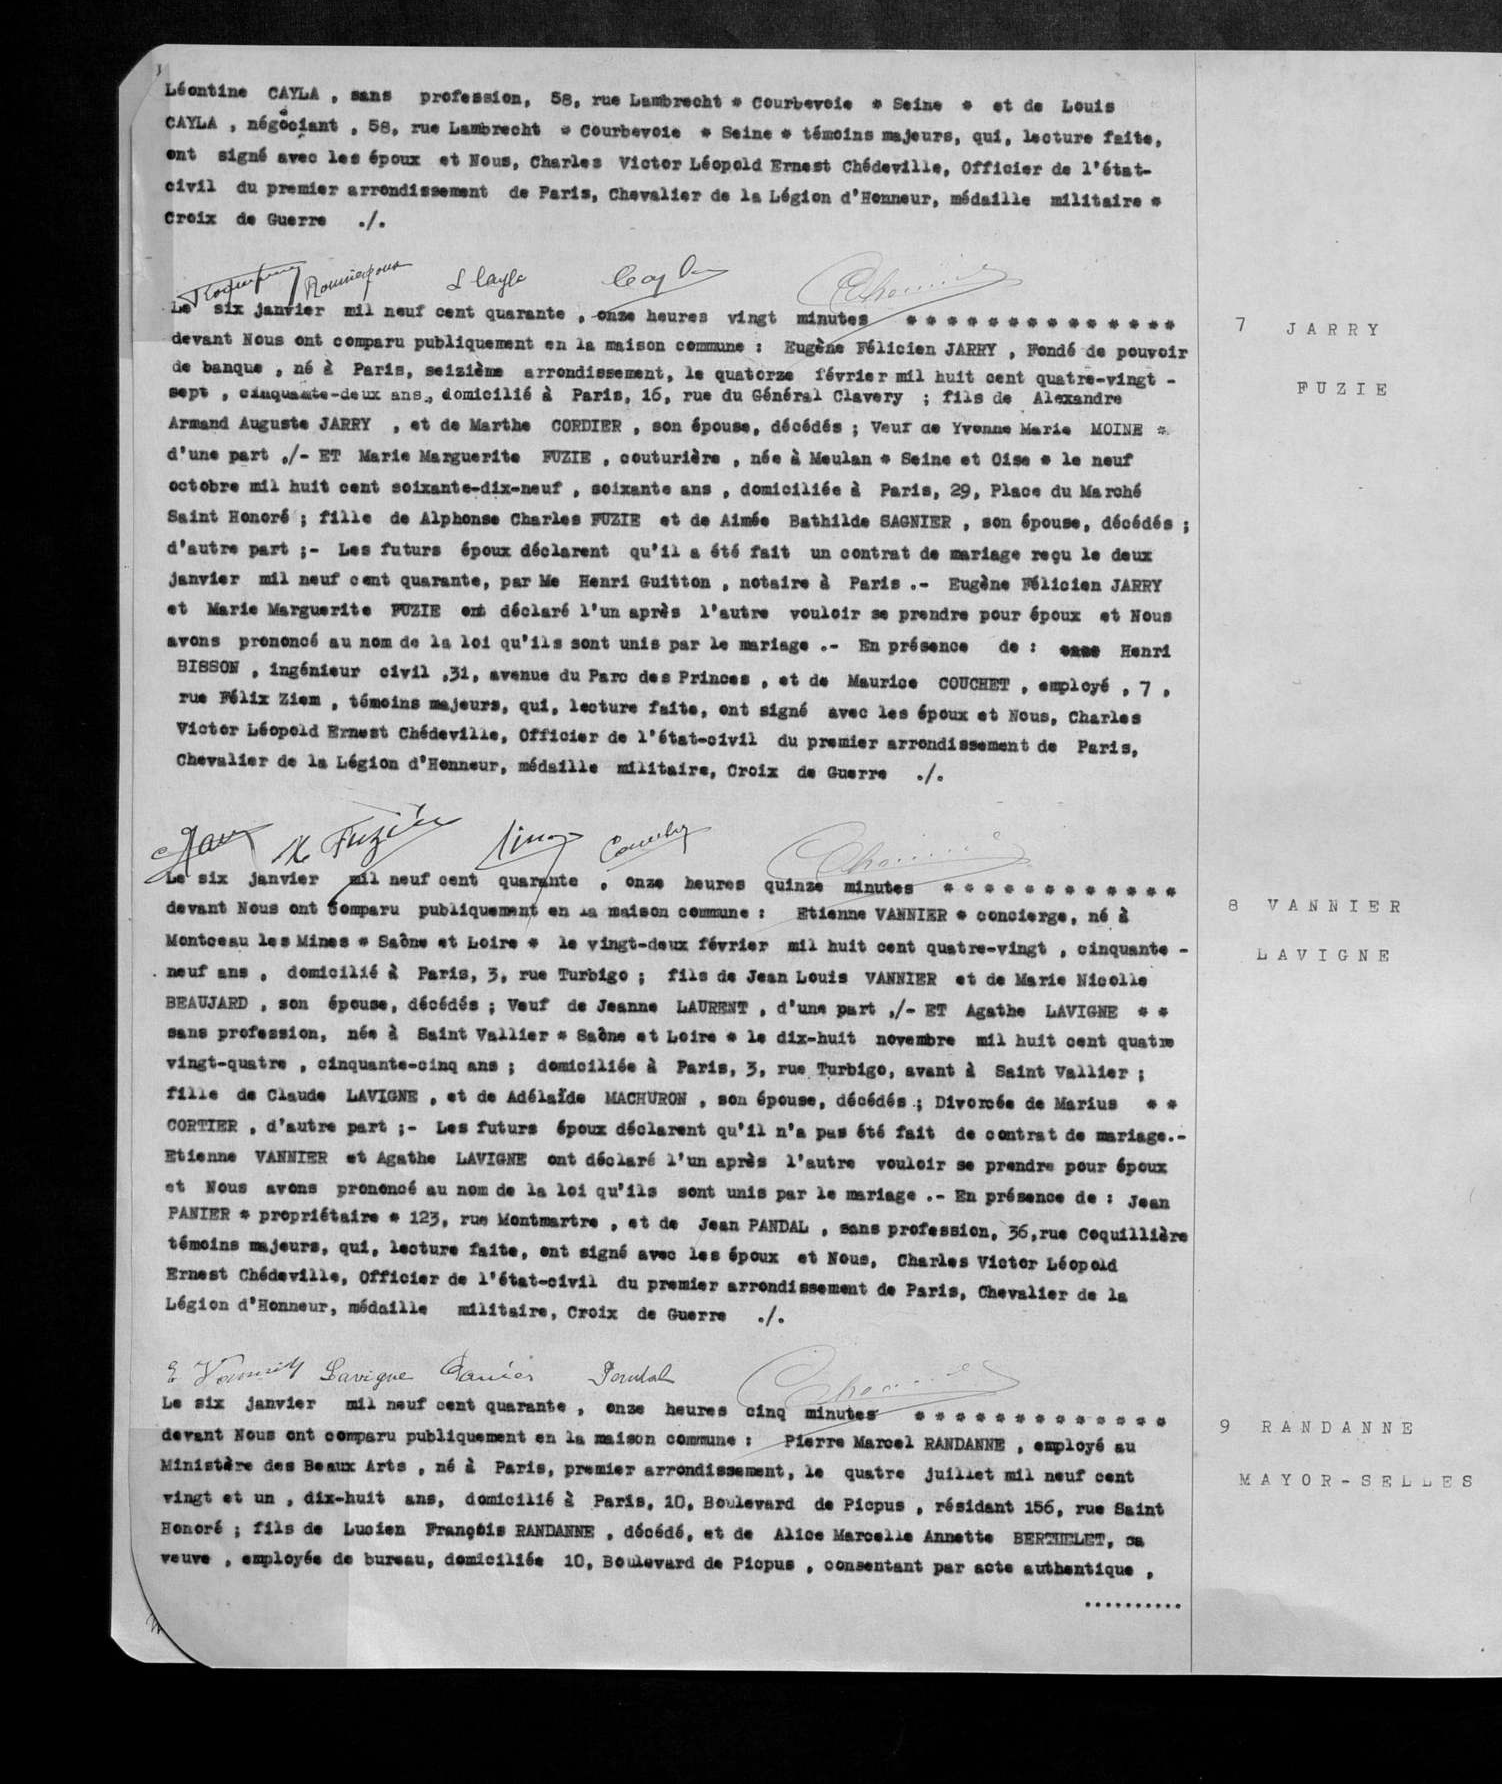Léontine CAYLA, sans profession, 58, rue Lambrecht * Courbevoie * Seine * et de Louis
CAYLA, négociant, 58, rue Lambrecht * Courbevoie * Seine * témoins majeurs, qui, lecture faite,
ont signé avec les époux et Nous, Charles Victor Léopolf Ernest Chédeville, Officier de l'état
civil du premier arrondissement de Paris, Chevalier de la Légion d'Honneur, médaille militaire *
Croix de Guerre ./.
Le six janvier mil neuf cent quarante, onze heures vingt minutes ****
devant Nous ont comparu publiquement en la maison commune: Eugène Félicien JARRY, Fondé de pouvoir
de banque, né à Paris, seizième arrondissement, le quatorze février mil huit cent quatre-vingt
sept, cinquante-deux ans, domicilié à Paris, 16 rue du Général Clavery; fils de Alexandre
Armand Auguste JARRY, et de Marthe CORDIER, son épouse, décédés; Veuf de Yvonne Marie MOINE *
d'une part ,/-ET Marie Marguerite FUZIE, couturière, née à Meulan * Seine et Oise * le neuf
octobre mil huit cent soixante-dix-neuf, soixante ans, domiciliée à Paris, 29, Place du Marché
Saint Honoré; fille de Alphone Charles FUZIE et de aimée Mathilde SAGNIER, son épouse, décédés;
d'autre part ;-Les futurs épouses déclarent qu'il a été fait un contrat de mariage reçu le deux
janvier mil neuf cent quarante, par Me Henri Guitton, notaire à Paris .-Eugène Félicien JARRY
et Marie Marguerite FUZIE ont déclaré l'un après l'autre vouloir se prendre pour époux et Nous
avons prononcé au nom de la loi qu'ils sont unis par le mariage .-En présence de: **** Henri
BISSON, ingénieur civil, 31 avenue du Parc des Princes, et de Maurice COUCHET, employé,
7, rue Félix Ziem, témoins majeurs, qui, lecture faite, ont signé avec les époux et Nous, Charles
Victor Léopold Ernest Chédeville, Officier de l'état-civil du premier arrondissement de Paris,
Chevalier de la Légion d'Honneur, médaille militaire, Croix de Guerre ./.
Le six janvier mil neuf cent quarante, onze heures quinze minutes ****
devant Nous ont comparu publiquement en la maison commune: Etienne WANNIER * concierge, né à
Montceau les Mines * Saône et Loire * le vingt-deux février mil huit cent quatre-vingt, cinquante-
neuf ans, domicilié à Paris, 3, rue Turbigo: fils de Jean Louis VANNIER et de Marie Nicelle
BEAUJARD, son épouse, décédés; Veuf de Jeanne LAURENT, d'une part ,/-ET Agathe LAVIGNE **
sans profession, née à Saint Vallier * Saône et Loire * le dix-huit novembre mil huit cent quatre
vingt-quatre, cinquante-cinq ans; domiciliée à Paris, 3, rue Turbigo, avant à Saint Vallier;
fille de Claude LAVIGNE, et de Adélaïde MACHRON, son épouse, décédés; Divorcée de Marius ***
CORTIER, d'autre part ;-Les futurs époux déclarent qu'il n'a pas été fait de contrat de mariage.
Etienne VANNIER et Agathe LAVIGNE ont déclaré l'un après l'autre vouloir se prendre pou époux
et Nous avons prononcé au nom de la loi qu'ils sont unis par le mariage .-En présence de: Jean
PANIER * propriétaire * 123, rue Montmartre, et de Jean PANDAL, sans profession, 36, rue Coquillière
témoins majeurs, qui, lecture faite, ont signé avec les époux et Nous, Charles Victor léopold
Ernest Chédeville, officier de l'état-civil du premier arrondissement de paris, Chevalier de la
Légion d'Honneur, médaille militaire, Croix de Guerre ./.
Le six janvier mil neuf cent quarante; onze heures cinq minutes ****
devant Nous ont comparu publiquement en la maison commune: Pierre Marcel RANDANNE employé au
Ministère des Beaux Arts, né à Paris, premier arrondissement, le quatre juillet mil neuf cent
vingt et un, dix-huit ans, domicilié à Paris, 10, Boulevard de Picpus, résidant 156, rue Saint
Honoré; fils de Lucien François RANDANNE, décédé, et de Alixe Marcelle Anette BERTHELET,
veuve, employée de bureau, domiciliée 10, Boulevard de Picpus, consentant par acte authentique,
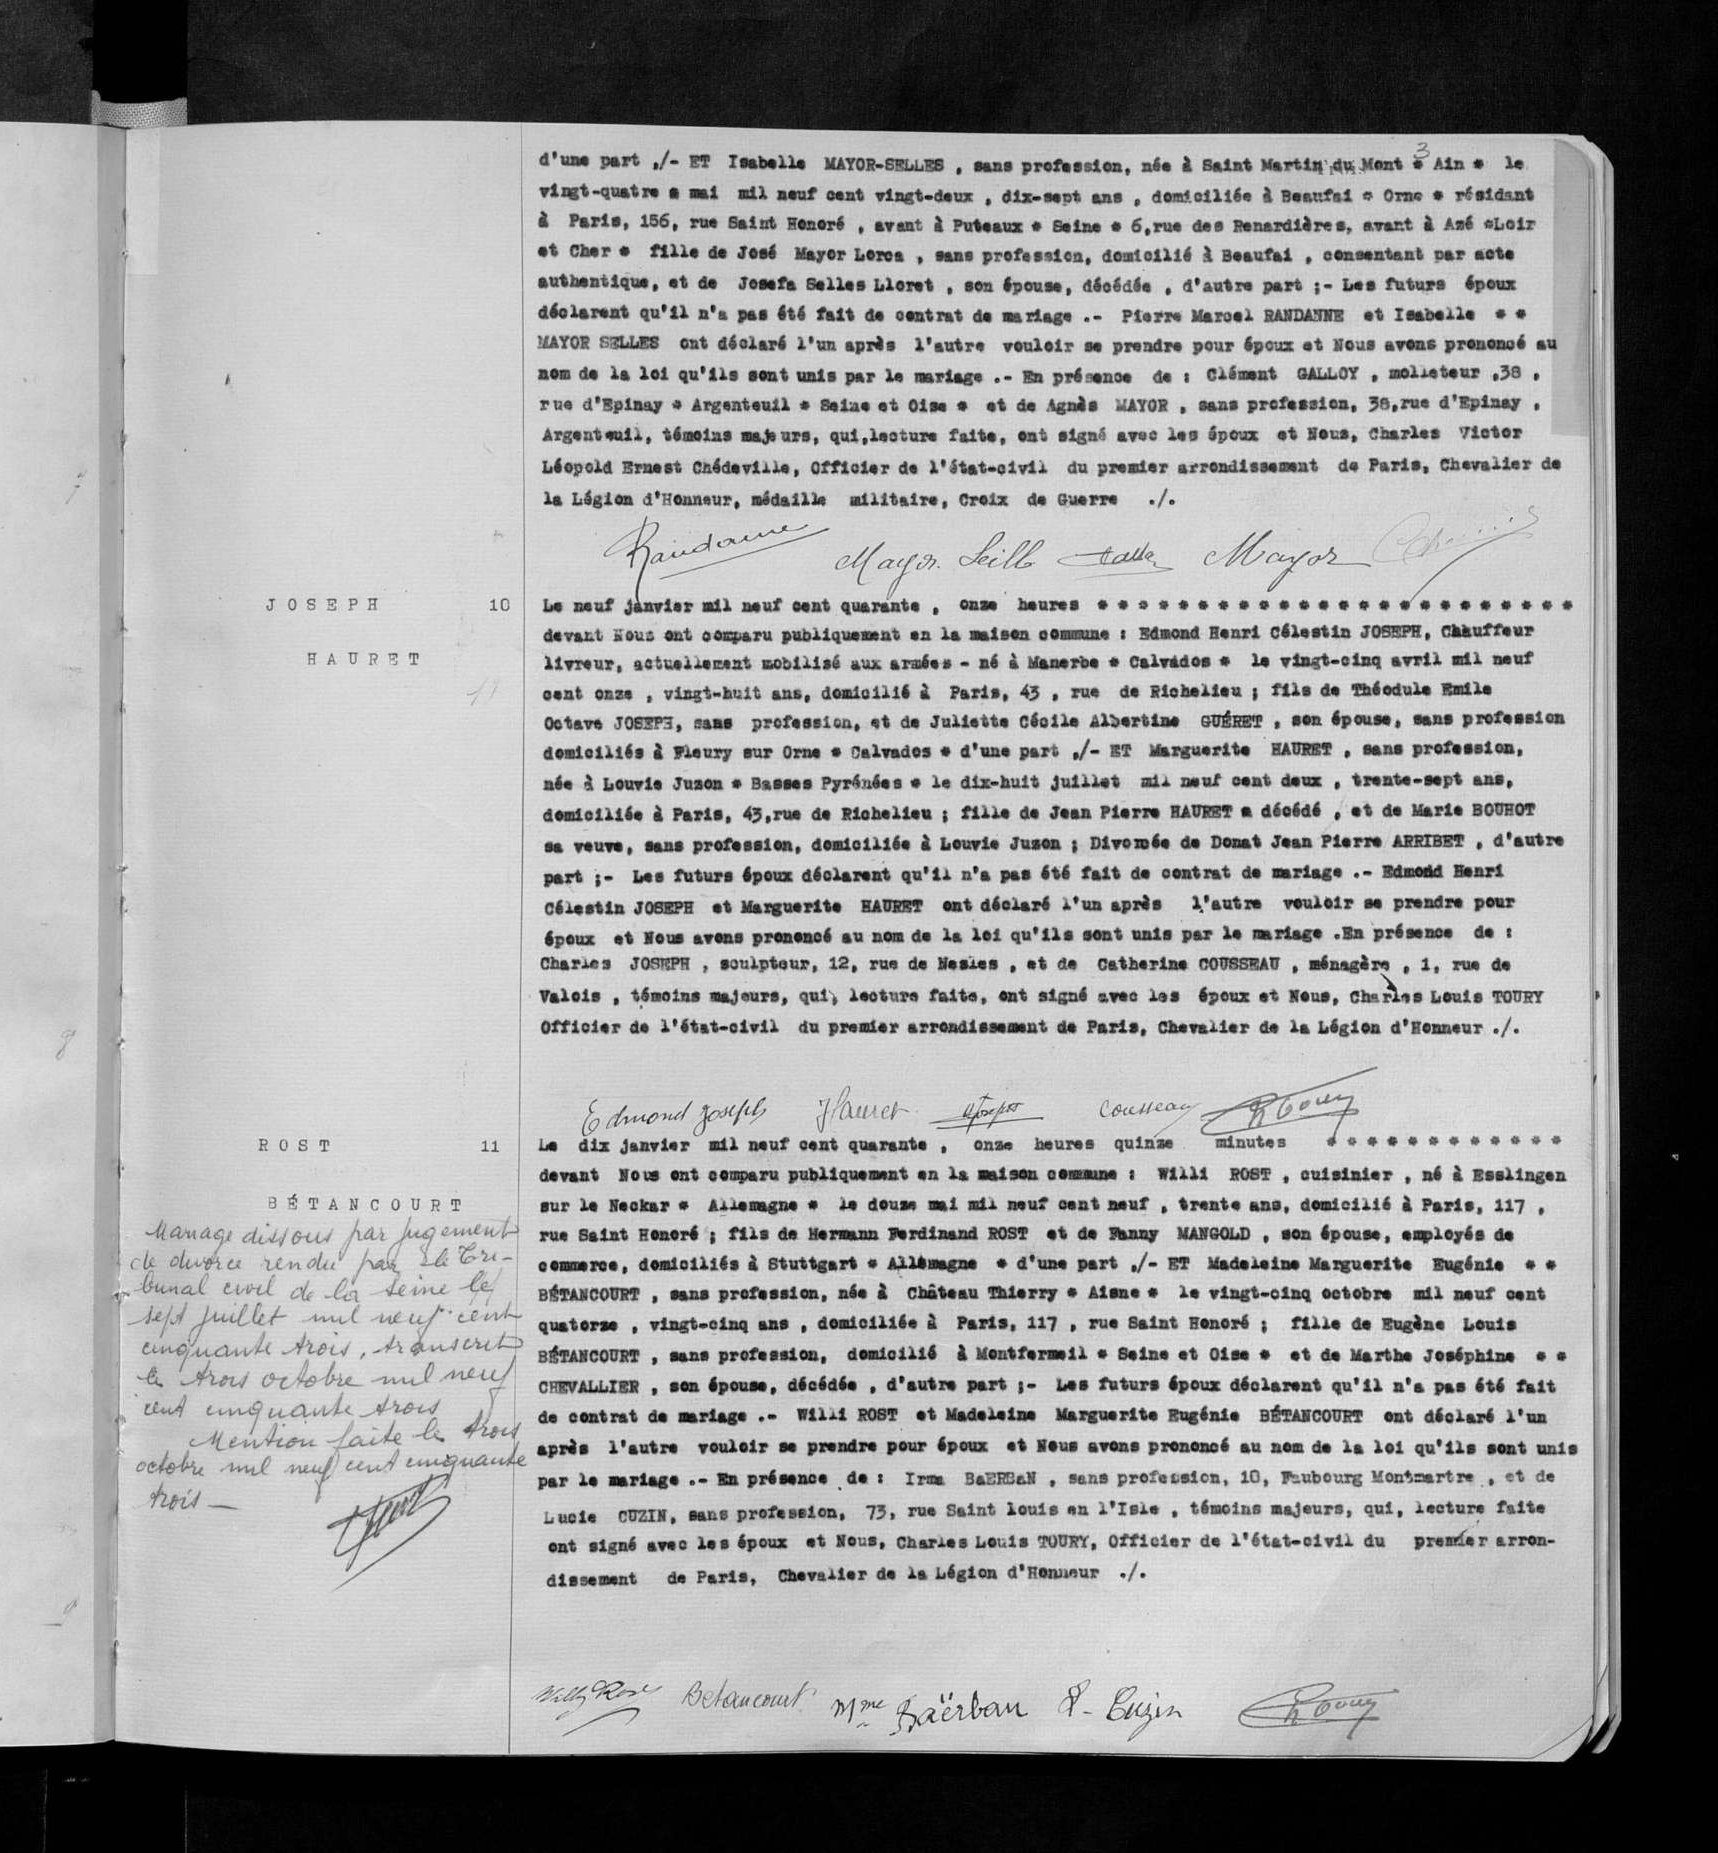d'une part, ET Isabelle MAYOR-SELLES, sans profession, née à Saint Martin du Mont * Ain *
le vingt-quatre * mai mil neuf cent vingt-deux, dix-sept ans, domiciliée à Beaufai * Orne * résidant
à Paris, 156, rue Saint Honoré, avant à Puteaux * Seine * 6, rue des Renardières, avant à Azé * Loir
et Cher * fille de José Mayor Lors, sans profession, domicilié à Beaufai, consentant par acte
authentique, et de Joseph Selles Lioret, son épouse, décédée, d'autre part;-Les futurs époux
déclarent qu'il n'a as été fait de contrat de mariage .-pierre Marcel RANDANTE et Isabelle **
MAYOR SELLES ont déclaré l'un après l'autre vouloir se prendre pour époux et Nous avons prononcé au
nom de la loi qu'ils sont unis par le mariage .-En présence de: Clément GALLOY, molleteur, 38,
rue d'Epinay * Argenteuil * Seine et Oise * et de Agnès MAYOR, sans profession, 38, rue d'Epinay,
Argenteuil, témoins majeurs, qui, lecture faite, ont signé avec les époux et Nous, Charles Victor
Léopolt ernest Chédeville, Officier de l'état-civil du premier arrondissement de Paris, Chevalier de
la Légion d'Honneur, médaille militaire, Croix de Guerre ./.
Le neuf janvier mil neuf cent quarante, onze heure ****
devant Nous ont comparu publiquement en la maison commune: Edmond Henri Celestin JOSEPH, Chauffeur
livreur, actuellement mobilisé aux armées-né à Manerbe * Calvados * le vingt-cinq avril mil neuf
cent onze, vingt-huit ans, domicilié à Paris, 43, rue de Richelieu; fils de Théodule Emile
Octave JOSEH, sans profession, et de Juliette Cécile Albertine GUERET, son épouse, sans profession
domiciliés à Fleury sur Orne * Calvados * d'une part ,/-ET Marguerite HAURET, sans profession,
née à Louvie Juson * Basses Pyrénées * le dix-huit juillet mil neuf cent deux, trente-sept ans,
domiciliée à Paris, 43 rue de Richelieu; fille de Jean Pierre HAURET * décédé, et de Marie BOUHOT
sa veuve, sans profession, domiciliée à Louvie Juson; Divorcée de Donat Jean Pierre ARRIBET, d'autre
part ;-Les futurs époux déclarent qu'il n'a pas été fait de contrat de mariage Edmond Henri
Célestin JOSEH et Marguerite HAURET ont déclaré l'un après l'autre vouloir se prendre pour
époux et Nous avons prononcé au nom de la Loi qu'ils sont unis par le mariage. En présence de:
Charles JOSEPH, sculpteur, 12, rue de Nesies, et de Catherine COUSSEAU, ménagère, 1, rue de
Valois, témoins majeurs, qui, lecture faite, ont signé avec les époux et Nous, Charles Louis TOURY
Officier de l'état-civil du premier arrondissement de Paris, Chevalier de la Légion d'Honneur ./.
Le dix janvier mil neuf cent quarante, onze heures quinze minutes ****
devant Nous ont comparu publiquement en la maison commune: Willi ROST, cuisinier, né à Esslingen
sur le Necker * Allemagne * le douze mai mil neuf cent neuf, trente ans domicilié à Paris, 117,
rue Saint Honoré; fils de Herman Ferdinand ROST et de Fanny MANGOLD, son épouse, employés de
commerce, domiciliés à Stuttgart * Allemagne * d'une part ;/-ET Madeleine Marguerite Eugénie **
BETANCOURT, sans profession, née à Château Thierry * Aisne * le vingt-cinq octobre mil neuf cent
quatorze, vingt-cinq ans, domiciliée à Paris 117, rue Saint Honoré; fille de Eugène Louis
BETANCOURT, sans profession, domicilié à Montfermeil * Seine et Oise * et de Marthe Joséphine **
CHEVALIER, son épouse, décédée, d'autre part ;-Les futurs époux déclarent qu'il n'a pas été fait
de contrat de mariage .-Willi ROST et Madeleine Marguerite Eugénie BETANCOURT ont déclaré l'un
après l'autre vouloir se prendre pour époux et Nous avons prononcé au nom de la lois qu'ils sont unis
par le mariage .-En présence de: Irme BaERSaN, sans profession, 10, Faubourg Montmartre, et de
Lucie CUZIN, sans profession, 73, rue Saint louis en l'Isle, témoins majeurs, qui, lecture faite
ont signé avec les époux et Nous, Charles Louis TOURY, Officier de l'état-civil du premier arron-
dissement de Paris, Chevalier de la Légion d'Honneur ./.